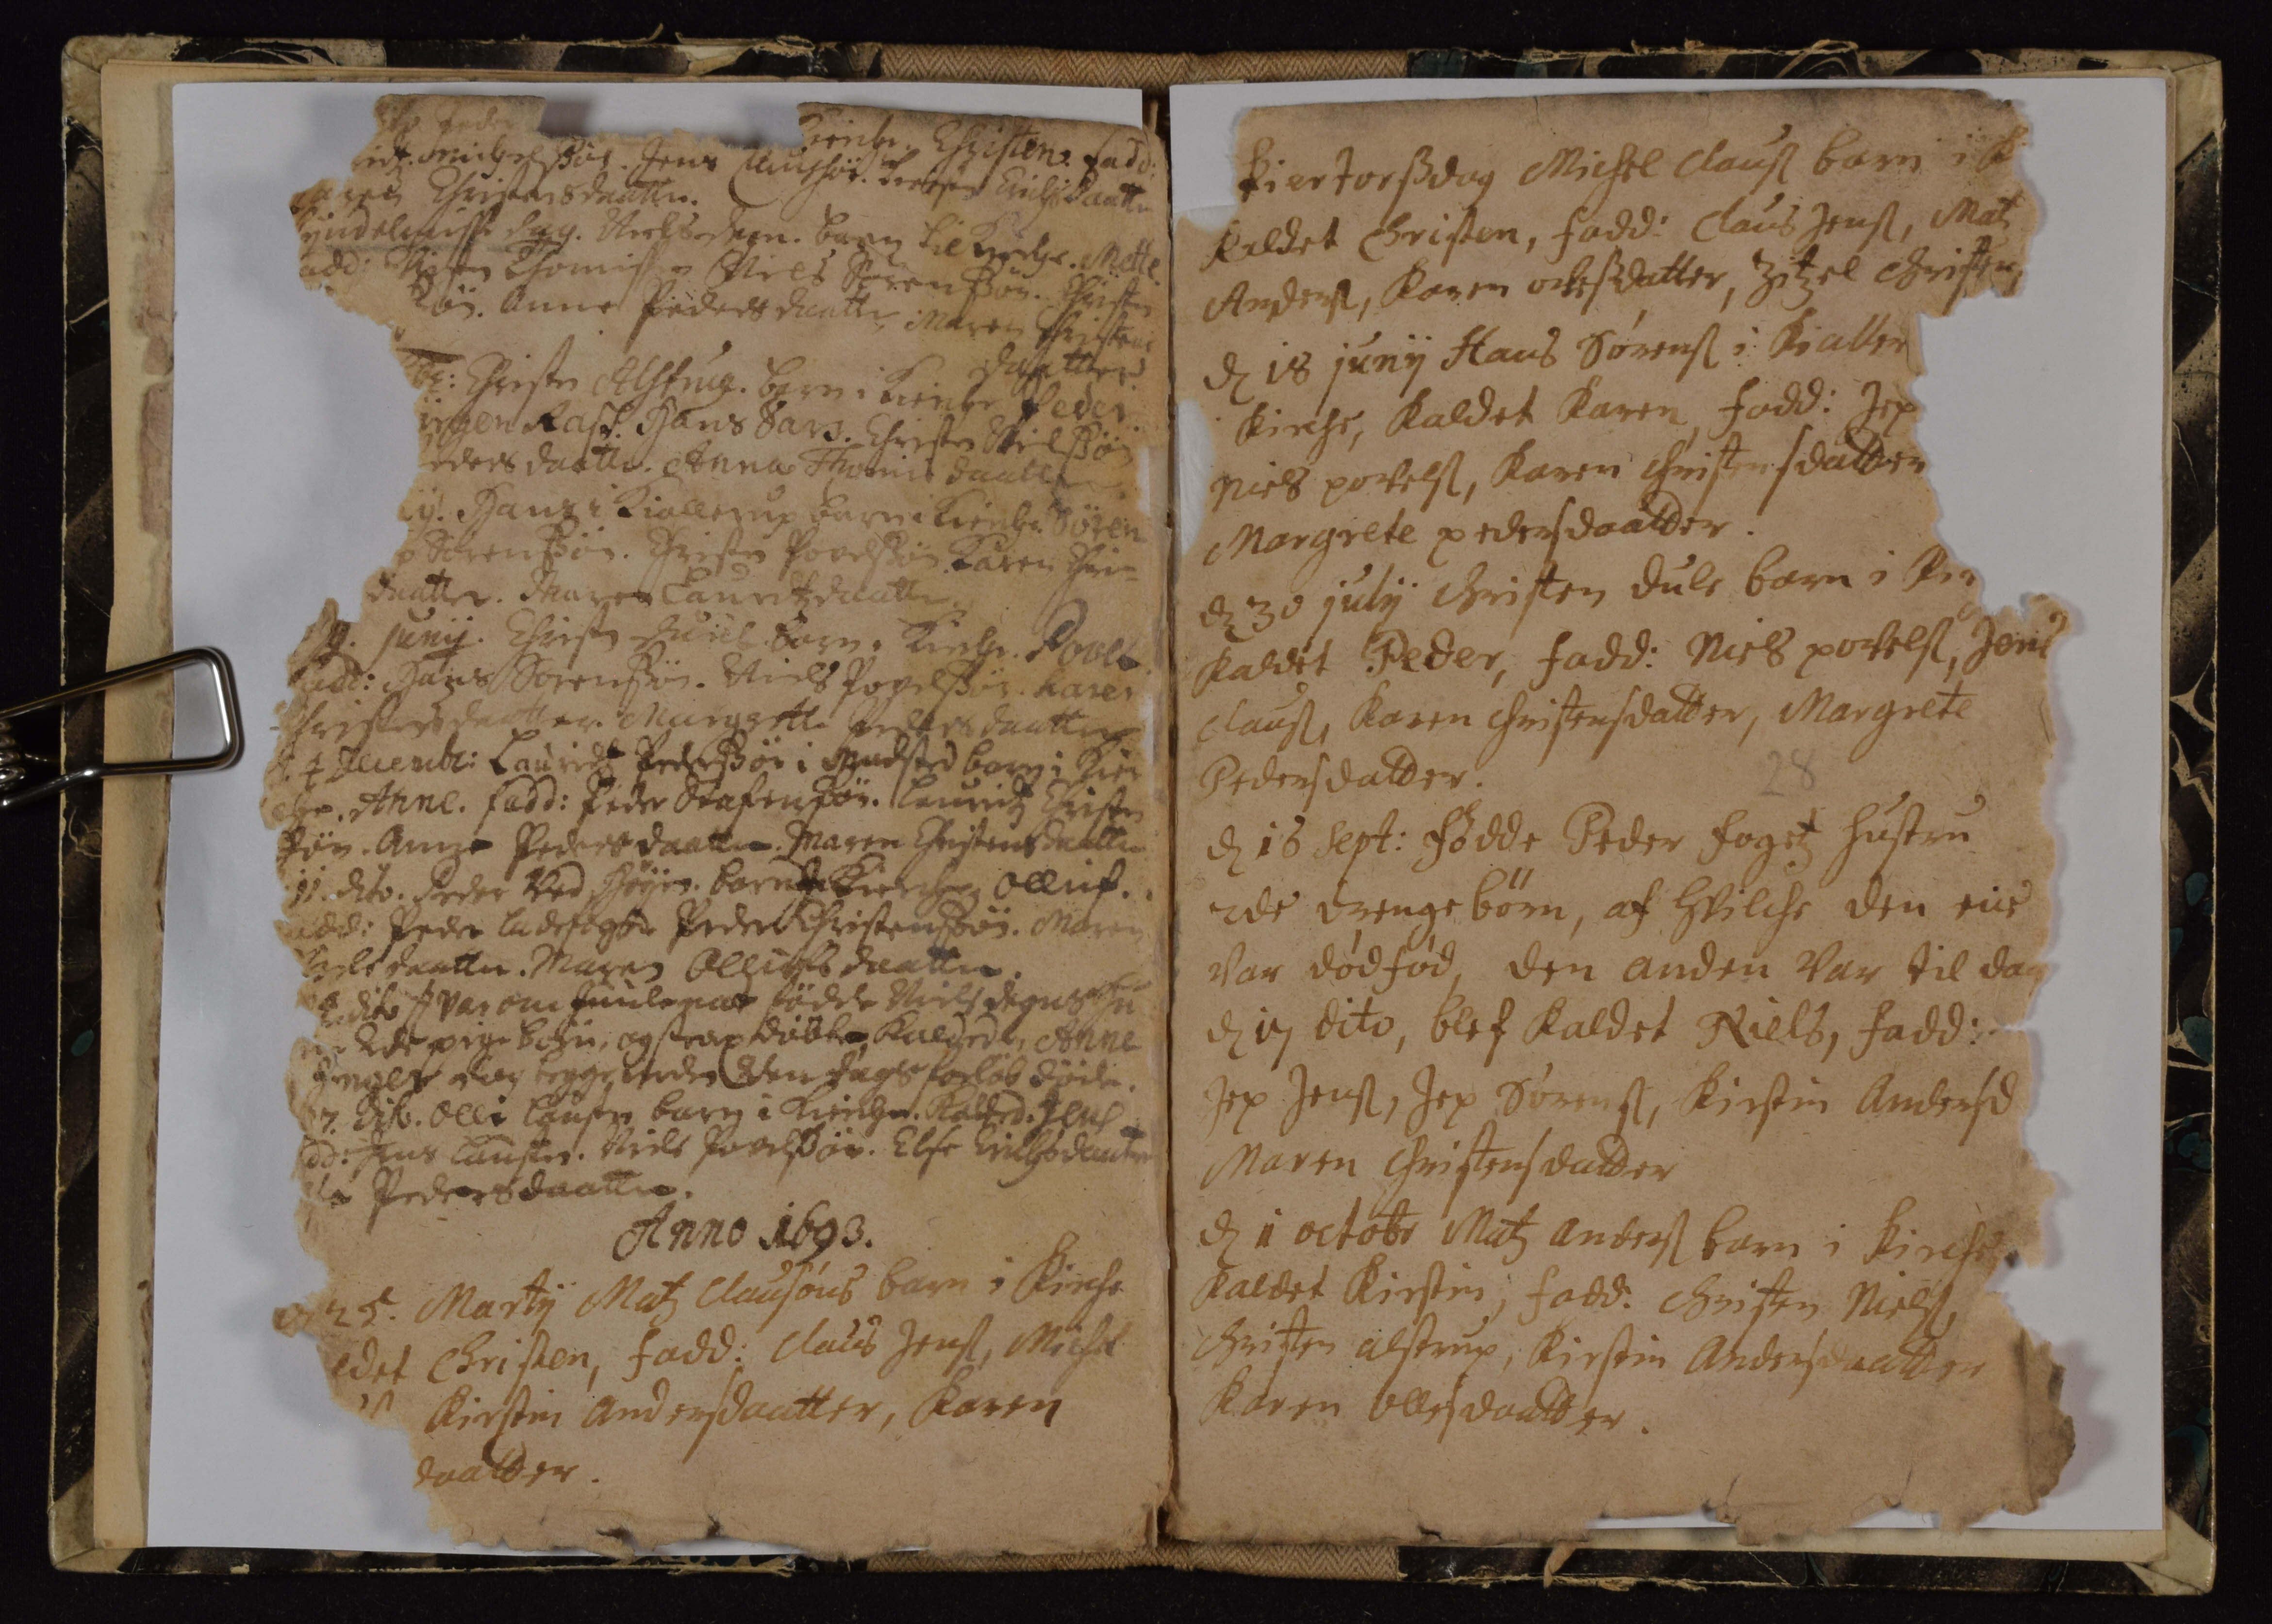## ##
Kirche Christens Fadd:
idz Michel ##. Jens Clausøn Kierten Erichsdaatter
## Christensdaatter.
Kyndelmisse dag. Niels degn. barn til Kirche. Mette
add: christen Thomissen Niels Sørenssøn. Chisten
##. Anne Peders datter Maren Christens
daatter.
br: Christen Alstrup barn i Lente Peder
##rgen Rask. Hans Sar##. Christen Nielssøn
eders dattter. Anna Thomis daater
##ij. Hans i Kiallerup barn i Lienhs Søren
## Sørenssøn. Christen Povelsøn, Karen Chri¬
daatter. Maren Lauridtzdaatter
9. Junij. Christen Niels barn i Kirche Povel
add: Hans Sørenssøn. Nils Povelssøn Karen
Christensdaatter Margrette Pedersdaatter
d 4 Decembr: Lauridtz Pederssøn i Madsted barn i Kir
che. Anne. Fadd: Peder Srafenssøn. Lauridtz Christen
søn. Anne Pedersdaater. Maren Christensdaatter.
11. dito. Peder ved Høyen. barn i Kirche Olluf.
add: Peder Ladefoget Peder Christenssøn. Maren
Nielsdaatter, Maren Olluffs daatter.
## var om Juulenat fødde Niels degns Hu
## 2de pige børn, strax døbte Kaldet, Anne
Inger dog begge ## ## dags forløb døde.
7. dcb. Olle Lausts barn i Kirche Kaldet. Jens
dd: Jens Lausten. Niels Povelsøn. Else Erichsdaater
## Pedersdaatter.
Anno 1693.
D. 25 Martij Matz Clausøns barn i Kirche
ldet Christen, Fadd. Claus Jens, Michel
Kirstin Andersdaatter, Karen
daatter.
Kiertorsdag Michel Claus barn i K
kaldet Christen, Fadd: Claus Jens, Matz
Anders, Karen ovssdatter, Zitzel Christen
d 18 Junij Hans Sørens i Kialler
Kirche, kaldet Karen, Fadd: Jep
Niels povels, Karen Christensdatter
Margrete pedersdaatter.
d 30 Julij christen dule barn i Kir
kaldet Peder, Fadd: Niels povels, Jens
Claus, Karen christensdatter, Margrete
Pedersdatter.
d 16 Sept. Fødde Peder Fogetz Hustru
2de drengebørn, af hvilhe den ene
var dødfød, den anden var til da
d 17 dito, blef kaldet Niels, Fadd:
Jep Jens, Jep Sørens, Kirstin Andersd
Maren Christensdatter
d 1 Octobr Matz Antes barn i Kirche
kaldet Kirstin; Fadd: Christen Niels
christten alstrup, Kirstin Andersdaatter
Karen ollesdaatter
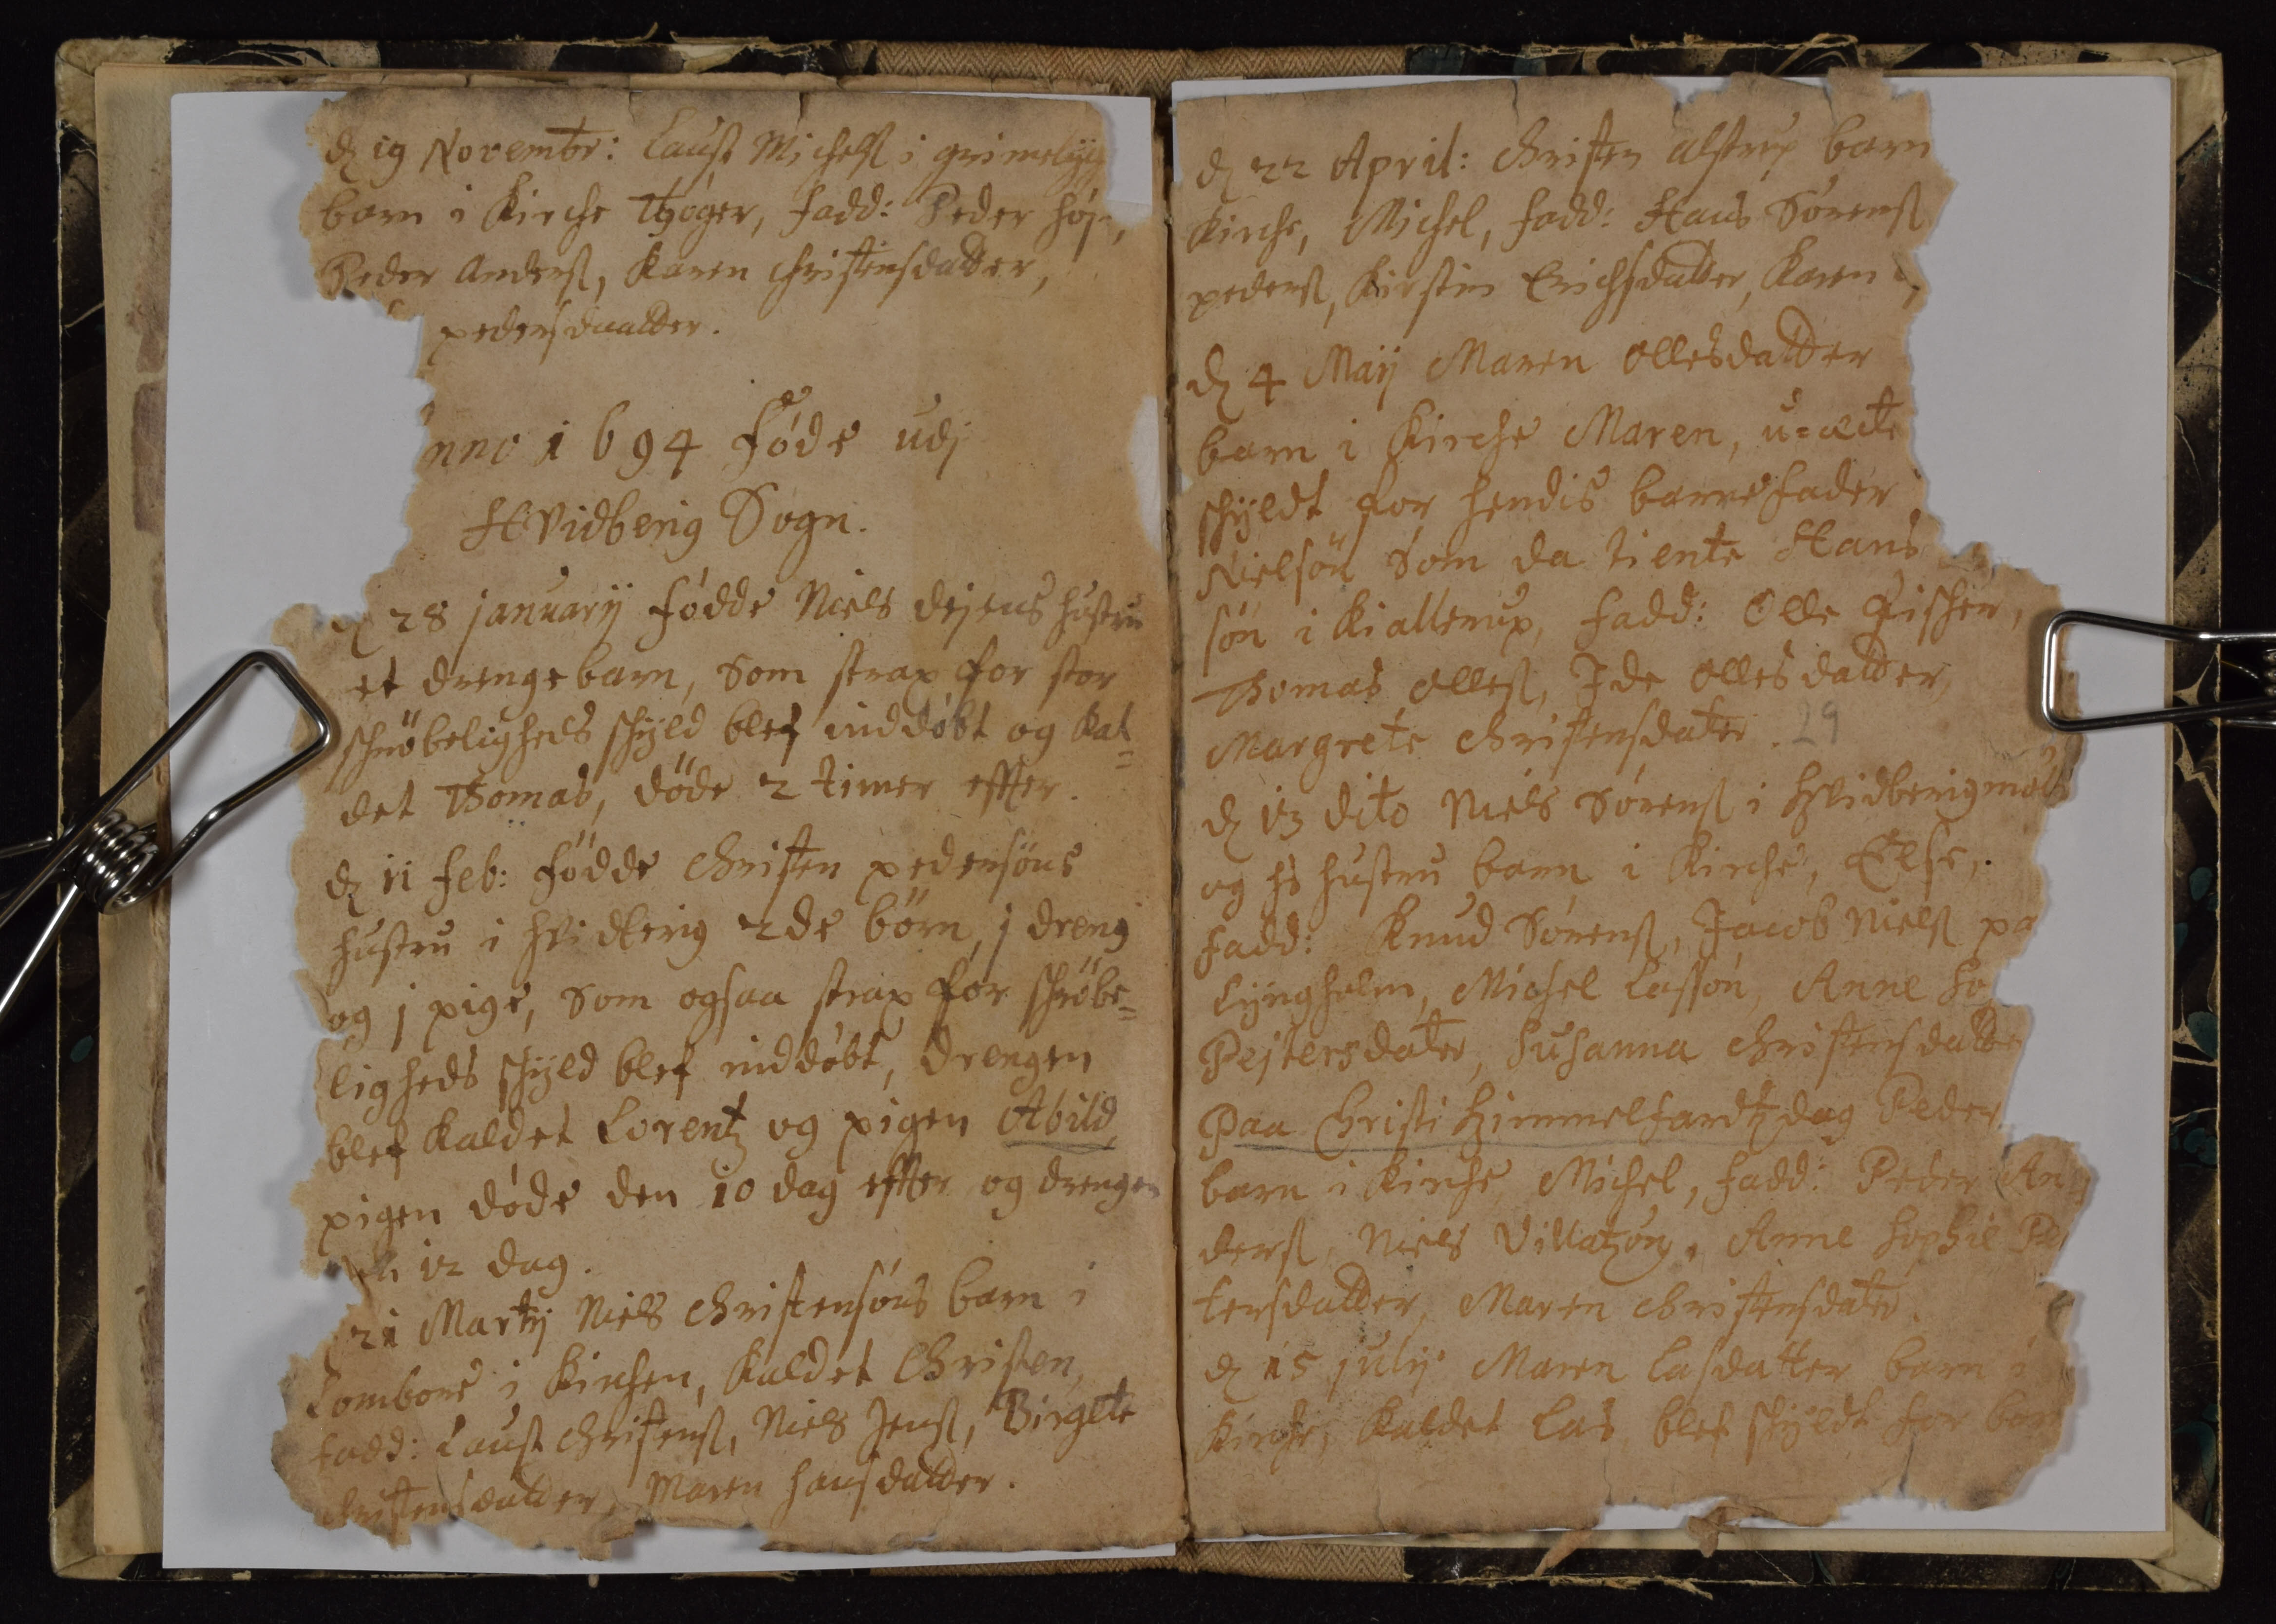d. 19 Novembr: Caus Michels i Grimelyche
barn i Kirche thøger, Fadd. Peder Høj
Peder Anders, Karen christensdatter
pedersdaatter
nno i 1694 føde udj
Hvidberig Sogn.
d 28 Januarij fødde Niels dejens hustru
et drengebarn, som strax for stor
shrøbeligheds shyld blef inddøbt og Kal¬
det Thomas, døde 2 timer effter.
d 11 Feb: fødde christen pedersøns
hustru i hvidberig 2de børn 1 dreng
og 1 pige, som ogsaa strax for skrøbe¬
ligheds shyld blef inddøbt, Drengen
blef kaldet Lorentz og pigen Abild
pigen døde den 10 Dag effter og drengen
## 12 Dag
21 Martij Niels christensøns barn i
Lombone i Kirchen, kaldet Christen
Fadd: Laust christens, Niels Jens, Birgete
##stens datter, Maren Hansdatter
d 22 April: Christen Alstrup barn
Kirche, Michel, Fadd: Hans Sørens
peders, Kirsten Erichsdatter Karen
d 4 Maij Maren Ollesdatter
barn i Kirche Maren, u=æcte
shyldt for hendis barne Fader
Nielsøn Som da tiente Hans
søn i Kiallerup, Fadd: Olle Fisher
Thomas Olles, Ide Olles datter
Margrete christensdater
d 13 dito Niels Søren i Hvidberig møl
og hs hustru barn i Kirche Else,
Fadd: Knud Sørens, Jacob Niels pa
Lyngholm, Michel Lassøn, Anne So
Pejtersdater, Susanna christensdatte
Paa Christi Hiemmelfardtz dag Peder
barn i Kirche Michel, Fadd: Peder ##
ders, Niels Villatzøn, Anne Sophie P##
tersdatter, Maren christensdater
d 15 Julij Maren Lasdatter barn i
Kirche kaldet Las blef shyldt for ba